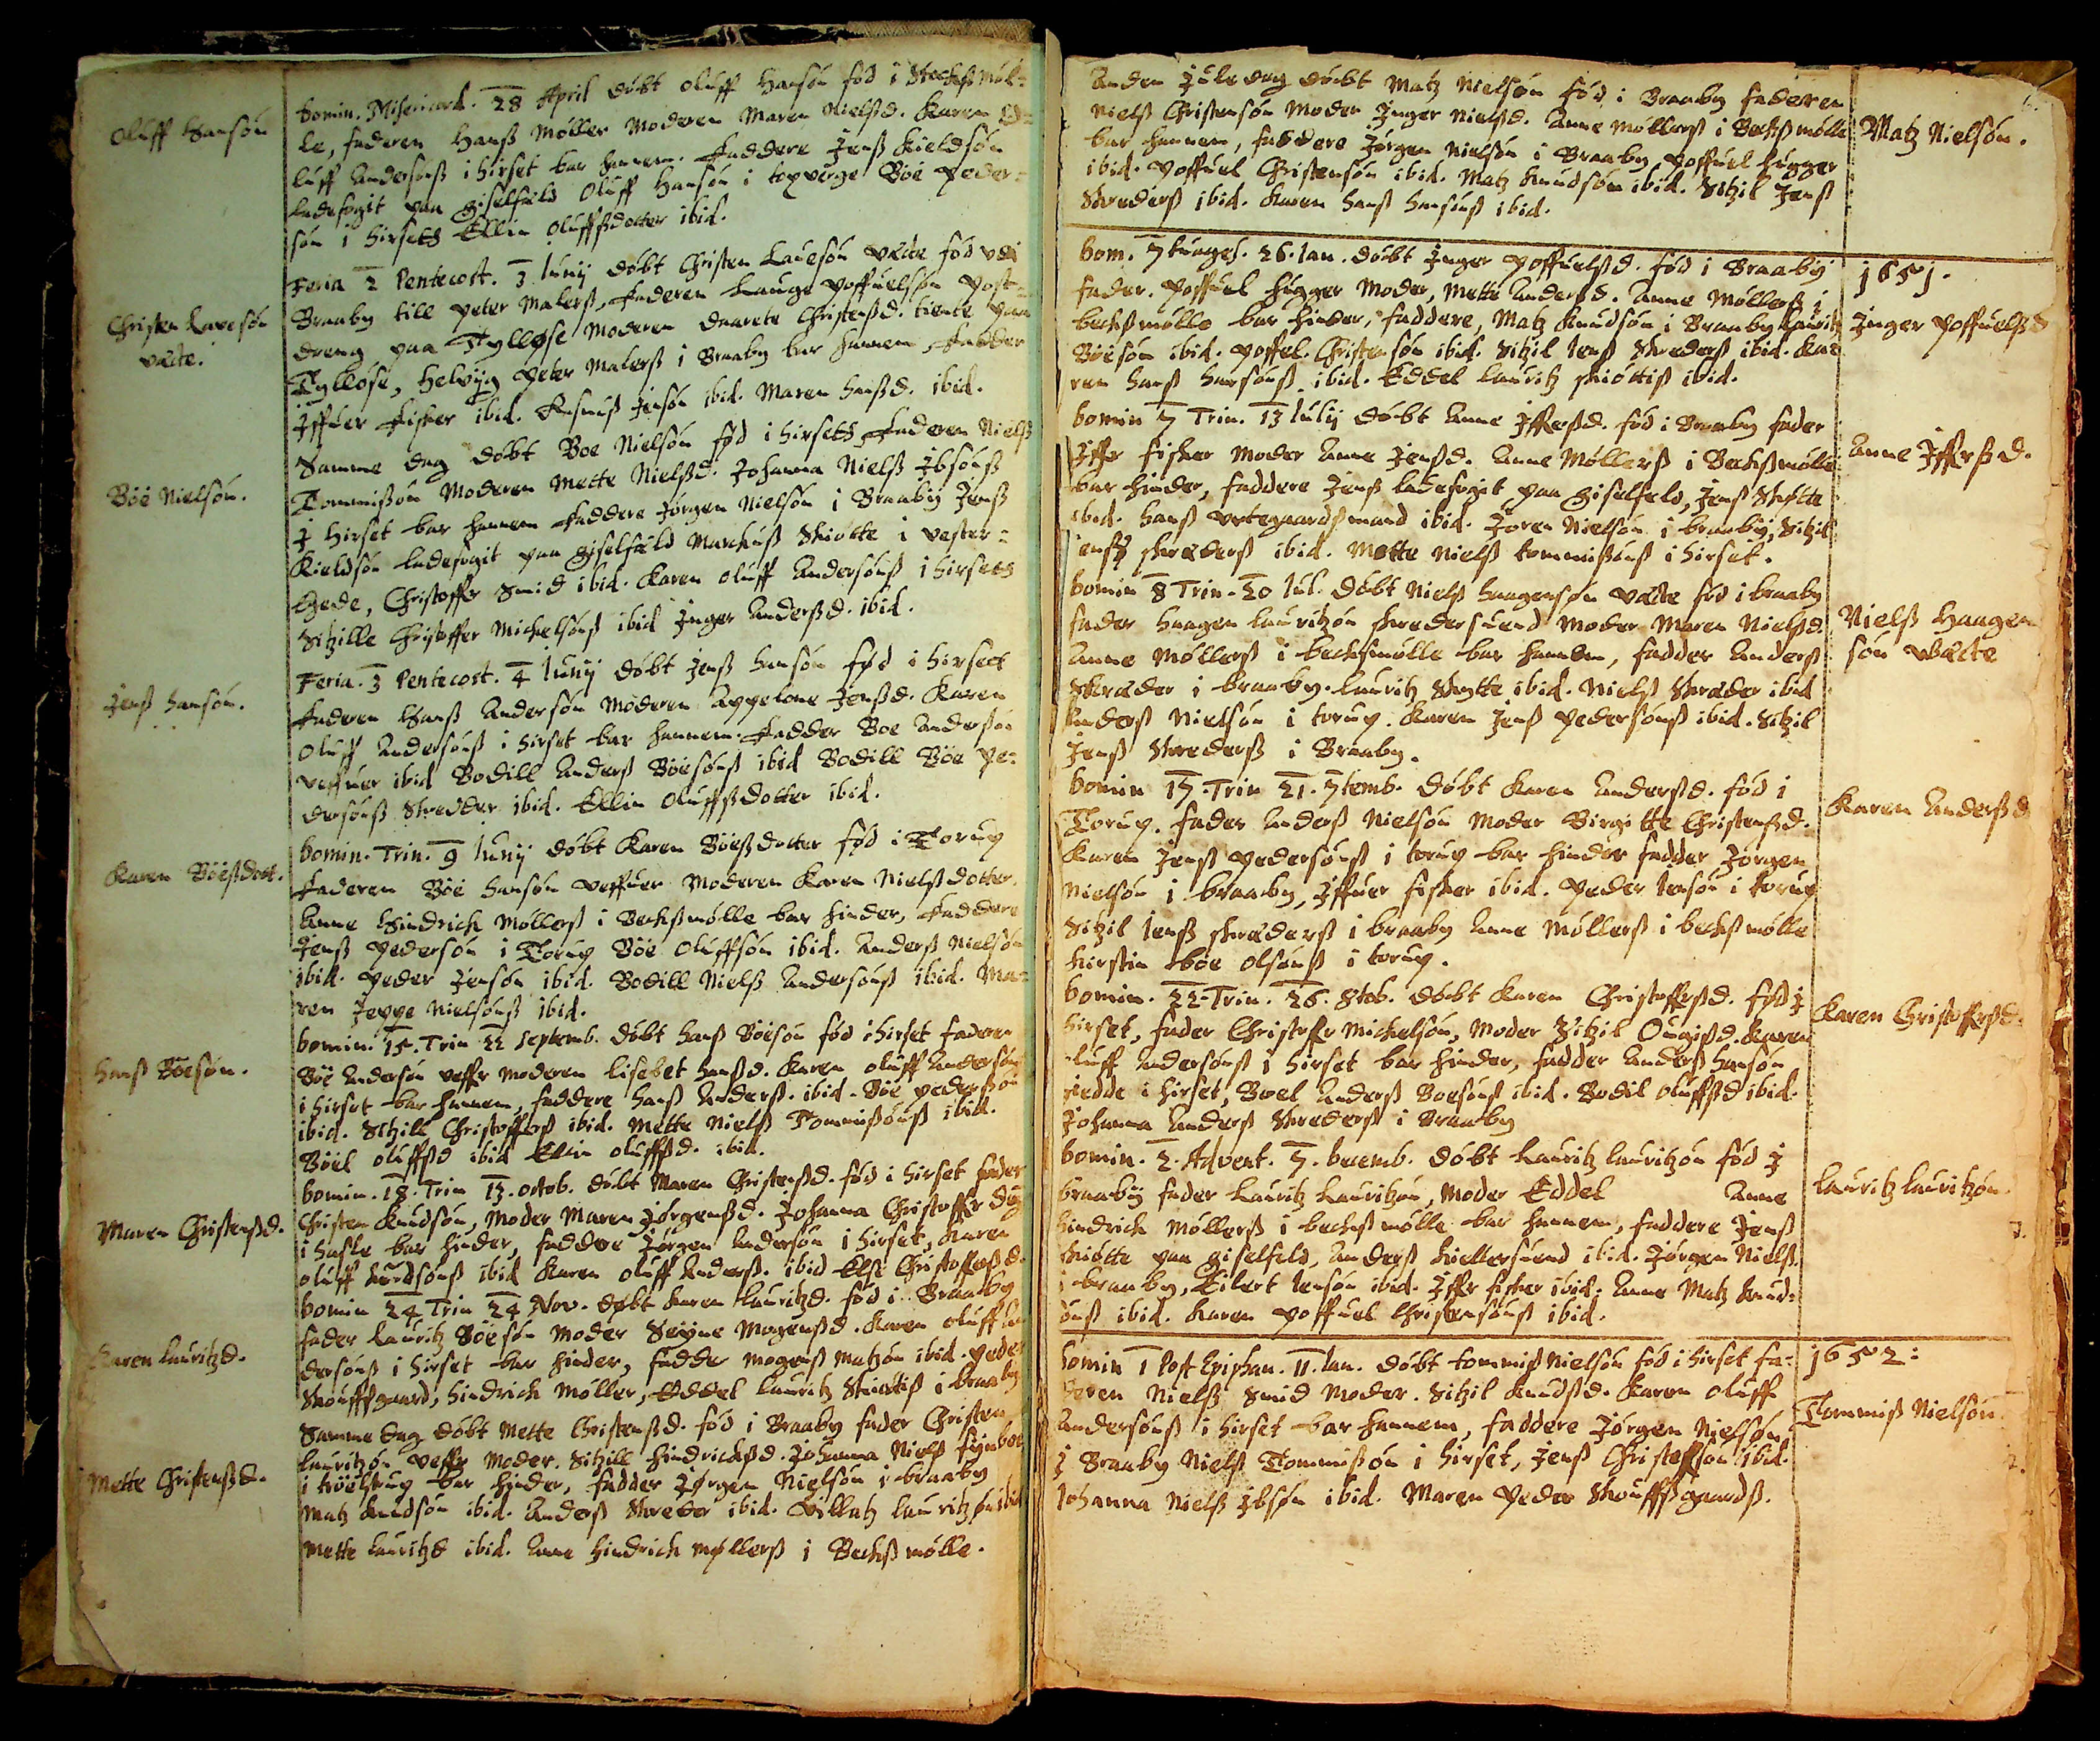Oluff Hansøn 
Domin. Misericord. 28 April døbt Oluff Hansøn fød i Stocksmøl¬
le, Faderen Hans Møller Moderen Maren Oluffsd. Karen O¬
luff Andersøns i Hirset bar hannem. Faddere Jens Kieldsøn
Ladefogit paa Giselfæld, Oluff Hansøn i Toxverge Boe Peder¬
søn i Hirset, Ellin Oluffsdotter ibid.
Christen Lauesøn 
Uæcte. 
Feria 2 Pentecost. 3. Juny døbt Christen Lauesøn uæcte fød udi
Braaby till Peder Malers, Faderen Lauge Poffuelsøn Post¬
dreng paa Tylløse. Moderen Daarete Christensd. tiente paa
Tylløse, Helvijg Peder Malers i Braaby bar hannem, Fadder
Jffuer Fisker ibid. Rasmus Jensøn ibid. Maren Hansd. ibid.
Boe Nielsøn 
Samme dag døbt Boe Nielsøn fød i Hirsett Faderen Niels
Tommisøn Moderen Mette Nielsd. Johanna Niels Jbsøns
J Hirset bar hannem Faddere Jørgen Nielsøn i Braaby Jens
Kieldsøn Ladefogit paa Giselfæld Markus Skiøtte i Vester¬
Egede, Christoffer Smid ibid. Karen Oluff Andersøns i Hirsett
Sitzille Christoffer Mickelsøns ibid. Inger Andersd. Ibid.
Jens Hansøn 
Feria. 3 Pentecost. 4. Juny døbt Jens Hansøn fød i Hirsett
Faderen Hans Andersøn Moderen Appelone Jensd. Karen
Oluff Andersøn i Hirset bar hannem. Fadder: Boe Andersøn
Veffuer ibid. Bodill Anders Boesøns ibid. Bodill Boe Pe¬
dersøns Skredder ibid. Ellin Oluffsdotter ibid.
Karen Boesdott. 
Domin. Trin. 9 Juny døbt Karen Boesdotter fød i Torup
Faderen Boe Hansøn veffuer. Moderen Karen Nielsdotter
Anne Hindrick Møllers i Becksmølle bar hinder, Faddere
Jens Pedersøn i Torup Boe Oluffsøn ibid. Anders Nielsøn
ibid. Peder Jensøn ibid. Bodill Niels Andersøns ibid. Ma¬
ren Jeppe Nielsøns ibid.
Hans Boesøn 
Domini 15. Trin 22 Septemb. døbt Hans Boesøn fød i Hirset. Faderen
Boe Andersøn Veffer Moderen Lisebet Hansd. Karen Oluff Andersøn
i Hirset bar hannem, Faddere Hans Anders. ibid. Boe Pedersøn
ibid. Sitzill Christoffers ibid. Mette Niels Tommisøns ibid.
Boel Oluffsd. ibid. Elin Oluffsd. ibid.
Maren Christensd. 
Domin. 18. Trin 13. Octob. døbt Maren Christensd. fød i Hirset Fader
Christen Knudsøn, Moder Maren Jørgensd. Johanna Christoffer Dug##
i Hasle bar hinder, Fadder Jørgen Andersøn i Hirset, Karen
Oluff Knudsøns ibid. Karen Oluff Anders. ibid Else Christoffersd.
Karen Lauritzd. 
Domin 24 Trin 24 Nov. døbt Karen Lauritzd. fød i Braaby.
Fader Lauritz Boesøn Moder Seigne Mogensd. Karen Oluff An
dersøns i Hirset bar hinder, Fadder Mogens Matzøn ibid. Peder
Skouffsgaard, Hindrick Møller, Eddel Lauritz Skiøtis i Braaby
Mette Christensd. 
Samme dag døbt Mette Christensd. fød i Braaby Fader Christen 
Lauritzøn Veffer Moder Sitzill Hindricksd. Johanna Niels Fynboe
i Tröëlstrup bar hinder, Fadder. Jørgen Nielsøn i Braaby
Matz Knudsøn ibid. Anders Skreder ibid. Villatz Lauritzøn ibid.
Mette Lauritzd ibid. Anne Hindrick Møllers i Becksmølle.
Anden Juledag døbt Matz Nielsøn fød i Braaby Faderen
Niels Christensøn Moderen Inger Nielsd. Anne Møllers i Becksmølle
bar hannem, Faddere Jørgen Nielsøn i Braaby, Poffuel Hugger
ibid. Poffuel Christensøn ibid. Matz Knudsøn ibid. Sitzil Jens
Skræders ibid. Karen Hans Hansøns ibid.
6.
Matz Nielsøn
Dom. 7tuages. 26. Jan. døbt Jnger Poffuelsd. fød i Braaby
Fader. Poffuel Hugger Moder, Mette Andersd. Anne Møllers i
Becksmølle bar hinder, Fadder, Matz Knudsøn i Braaby Lauritz
Boesøn ibid. Poffuel Christensøn ibid. Sitzil Jens Skræders ibid. Kar
ren Hans Hansøns ibid. Eddel Lauritz Skiøtis ibid.
1651. 
Inge Poffuelsd. 
Dom. 7. Trin 13. July døbt Anne Jffersd fød i Braaby Fader
Jffer Fisker, Moder Anne Jensd. Anne Møllers i Becksmølle
bar hinder, Faddere: Jens Ladefoget paa Giselfæld, Jens Skøtte
ibid. Hans Urtegaardsmand ibid. Jøren Nielsøn i Braaby, Sitzil
Jens Skræders ibid. Mætte Niels tommisøns i Hirset.
Anne Iffersd.
Domin 8 Trin. 20 Jul. døbt Niels Haagesøn uæcte fød i Braaby
Fader Haagen Lauritzøn Skrædersvend, Moder Maren Nielsd.
Anne Møllers i Becksmølle bar hannem, Fadder Anders
Skræder i Braaby. Lauritz Skytte ibid. Niels Skræder ibid
Anders Nielsøn i torup. Karen Jens Pedersøns ibid. Sitzil
Jens Skræders i Braaby.
Niels Haage¬
søn Uæcte
Domin. 17. Trin 7temb. døbt Karen Andersd. fød i
Torup. Fader Anders Nielsøn Moder Birgitte Christensd.
Karen Jens Pedersøns i torup bar hinder Fadder: Jørgen
Nielsøn i Braaby, Jffuer Fisker ibid. Peder Jensøn i Torup
Sitzil Jens Skrædders i Braaby Anne Møllers i becksmølle
Kirstin Boe Olsøns i torup.
Karen Andersd.
Domin. 22. Trin 26. 8tob. døbt Karen Christoffersd. fød J
Hirset, Fader Christoffe Mickelsøn, Moder Sitzil Ougisd. Karen
Oluff Andersøns i Hirset bar hinder, Fadder: Anders Hansøn
Giedde i Hirset. Boel Anders Boesøns ibid. Bodil Oluffsd. ibid.
Johanna Anders Skreders i Braaby
Karen Christoffersd.
Domin. 2. Advent 7. Decemb. døbt Lauritz Lauritzøn fød J
Braaby Fader Lauritz Lauritzøn, Moder Eddel
Anne
Hindrick Møllers i becksmølle bar hannem, Faddere Jens
Skiøtte paa Giselfeld, Anders Kieldersvend ibid. Jørgen Niels.
i Braaby. Eilert Jensøn ibid. Jffer Fisker ibid. Anne Matz Knud¬
søns ibid. Karen Poffuel Christensøns ibid.
Lauritz Lauritzøn
Domin 1 Post Epiphan. 11. Jan. døbt Tommis Nielsøn fød i Hirset Fa¬
deren Niels Smid Moder. Sitzil Knudsd. Karen Oluff 
Andersøns i Hirset bar hannem, Faddere Jørgen Nielsøn
J Braaby Niels Tommisøn i Hirset, Jens Christoffsøn ibid.
Johanna Niels Jbsøn ibid. Maren Peder Skouffsgaards.
1652:
Tommis Nielsøn